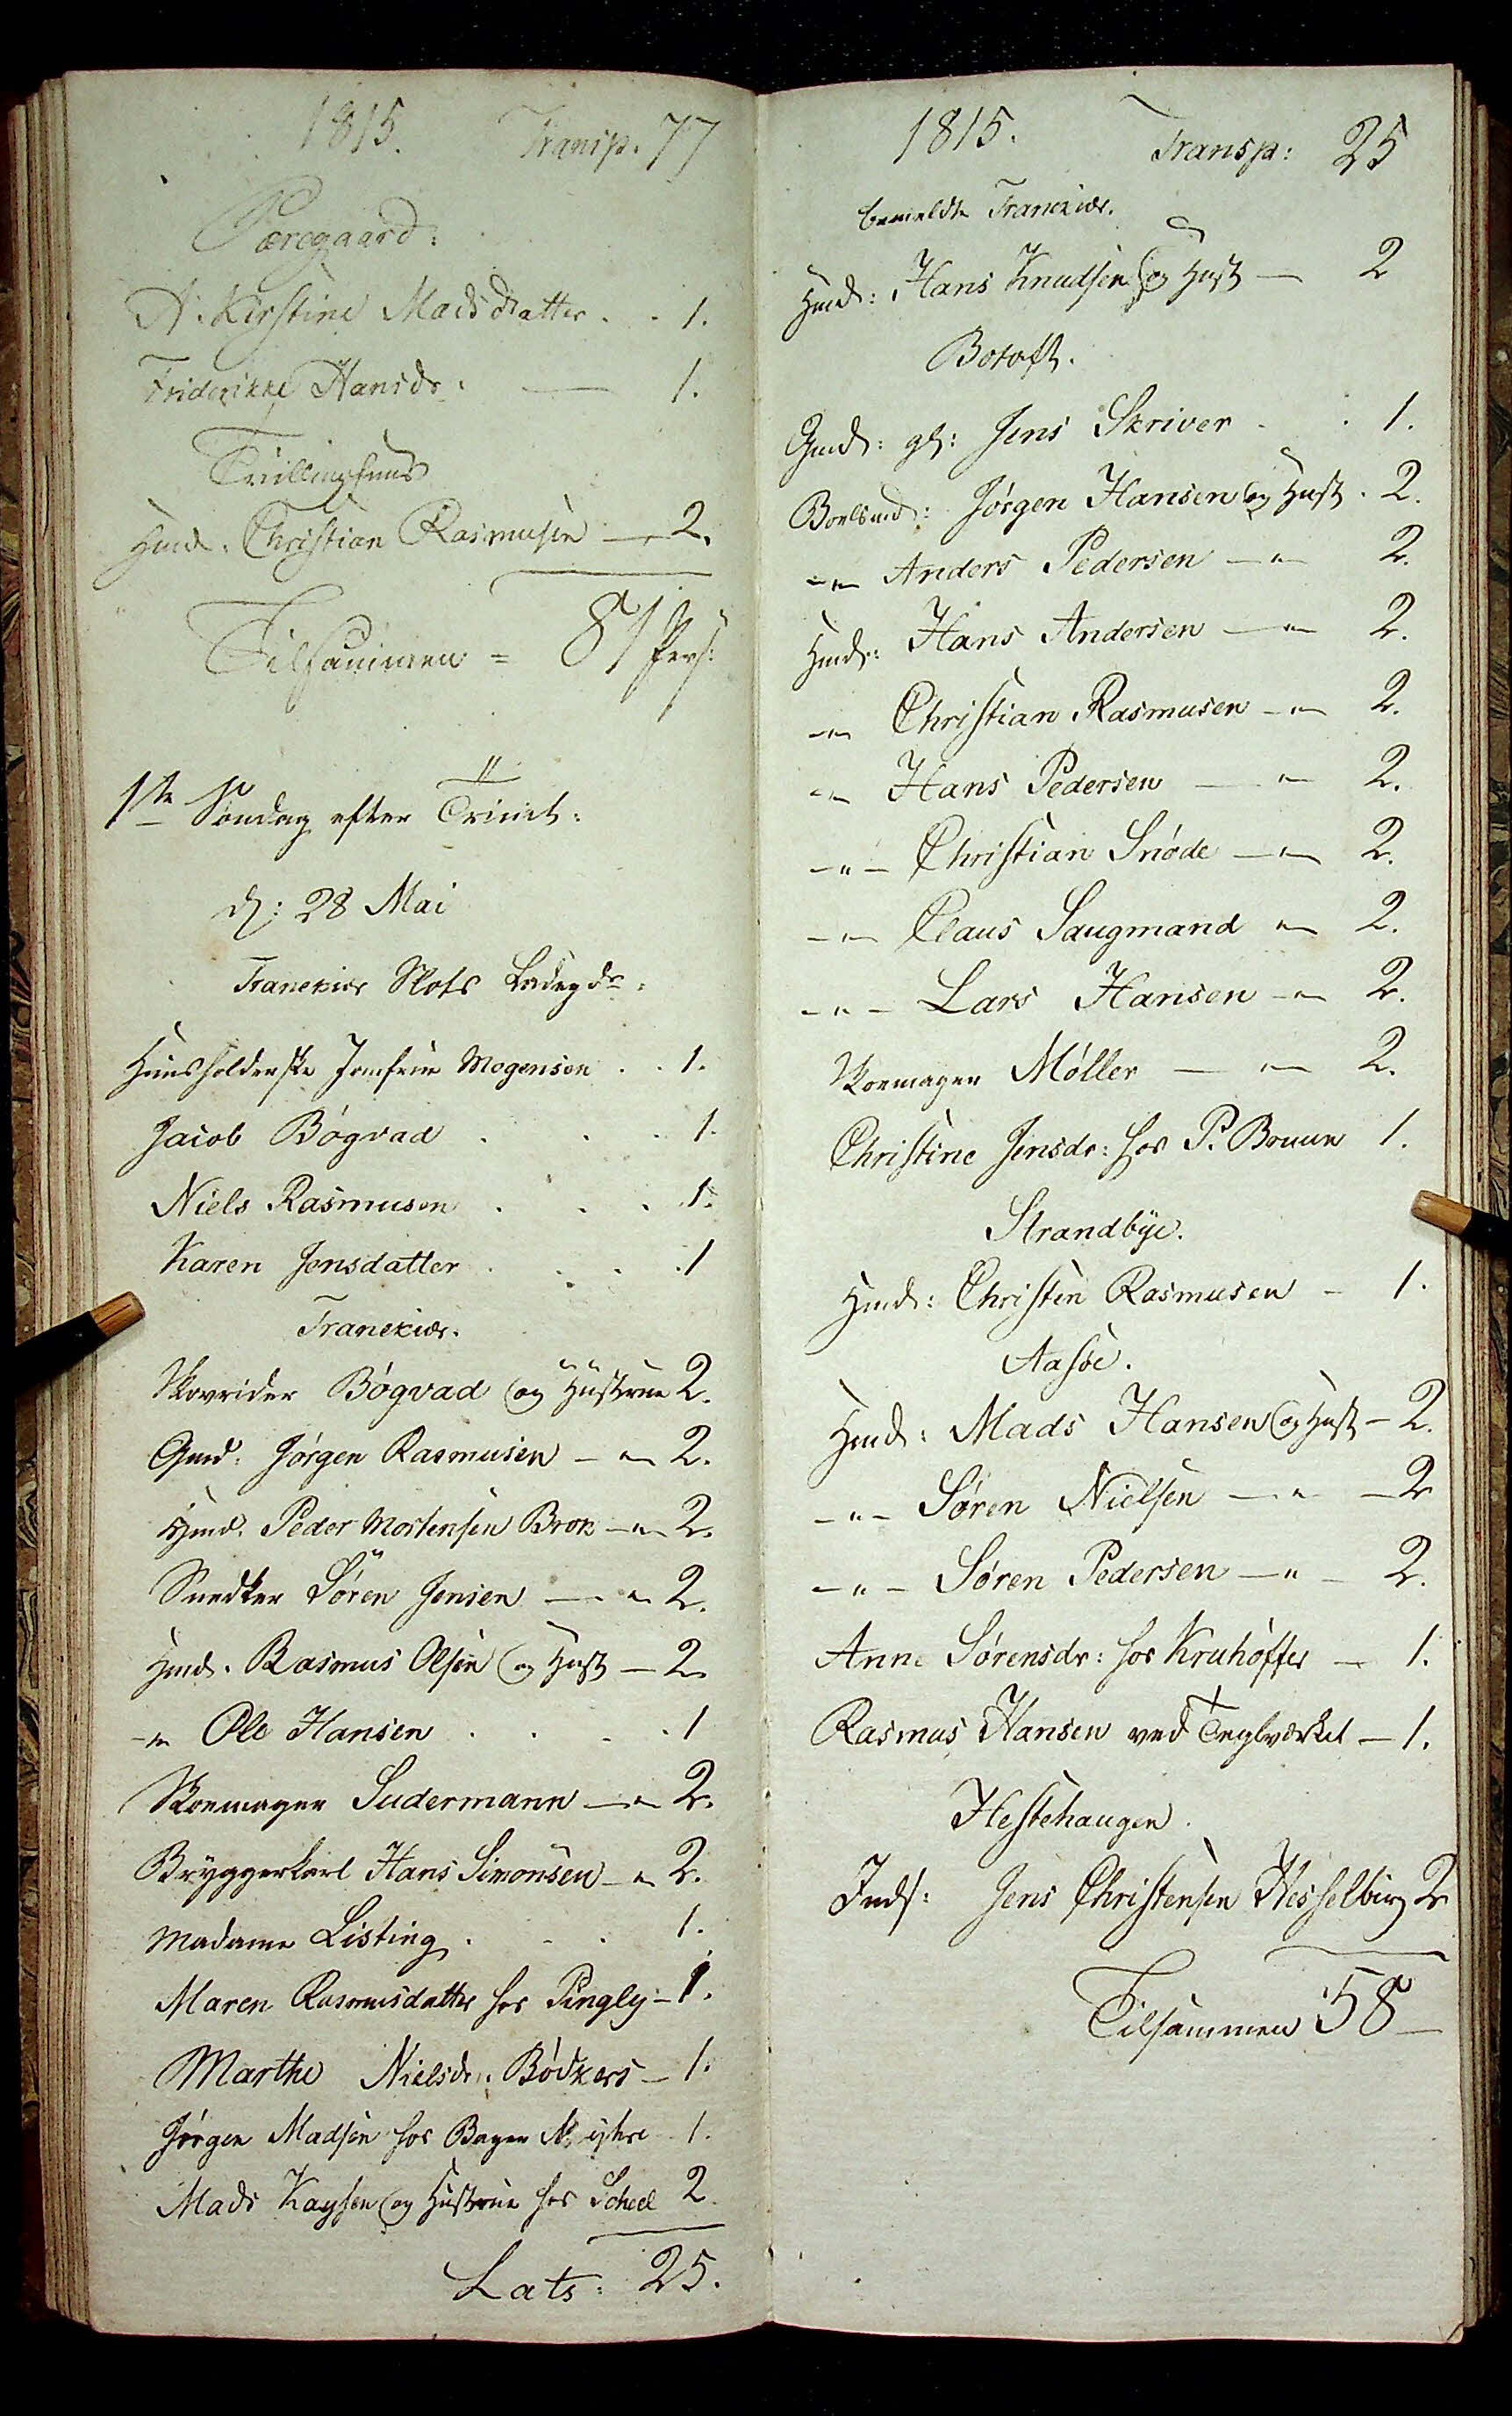1815.
Tranp: 77
Pæregaard:
A. Kirstine Madsdatter . . 1.
Friderikke Hansdr . - 1.
Tvillinghuus
Hmd: Christian Rasmusen - 2.
Tilsammen = 81 Pers:
1ste Søndag efter Trinit:
d: 28 Mai
Tranekiær Slots Ladagdr
Huusholderske Jomfrue Mogensen . . 1.
Jacob Bøgvad . . . 1.
Niels Rasmusen . . . 1.
Karen Jensdatter . . . . 1
Tranekiær.
Skovrider Bøgvad og Hustrue 2.
Gmd: Jørgen Rasmusen -"- 2.
Hmd. Peder Mortensen Brok -"- 2.
Snedker Søren Jensen -"- 2.
Hmd. Rasmus Olsen og Hust - 2.
-"- Ole Hansen . . . . 1
Skoemager Sudermann -"- 2.
Bryggerkarl Hans Simonsens -"- 2.
Madame Listing . . . 1.
Maren Rasmusdatter hos Pingly - 1.
Marthe Nielsdr Bødkers - 1.
Jørgen Madsen hos Bager Myhre . 1.
Mads Kaysen og Hustrue hos PScheel 2.
Lats: 25.
1815.
Transp: 25
bemeldte Tranekiær.
Hmd: Hans Knudsen og Hust - 2
Botoft.
Gmd: gl: Jens Skriver . .1.
Boelsmd: Jørgen Hansens og Hust . 2.
-"- Anders Pedersen -"- 2.
Hmd: Hans Andersen -"- 2.
-"- Christian Rasmusen -"- 2.
-"- Hans Pedersen -"- 2.
-"- Christian Snøde -"- 2.
-"- Claus Saugmand -"- 2.
-"- Lars Hansen -"- 2.
Skomager Møller -"- 2.
Christine Jensen hos P. Bruun 1.
Strandbye.
Hmd: Christen Rasmusen - 1
Aasøe.
Hmd: Mads Hansens og Hust - 2.
-"- Søren Nielsen -"- 2
-"- Søren Pedersen -"- 2.
Anne Sørensdr: hos Kruhøffer - 1.
Rasmus Hansen ved Teglværket - 1.
Hestehaugen
Inds: Jens Christensen Hesselbirg 2
Tilsammen 58-
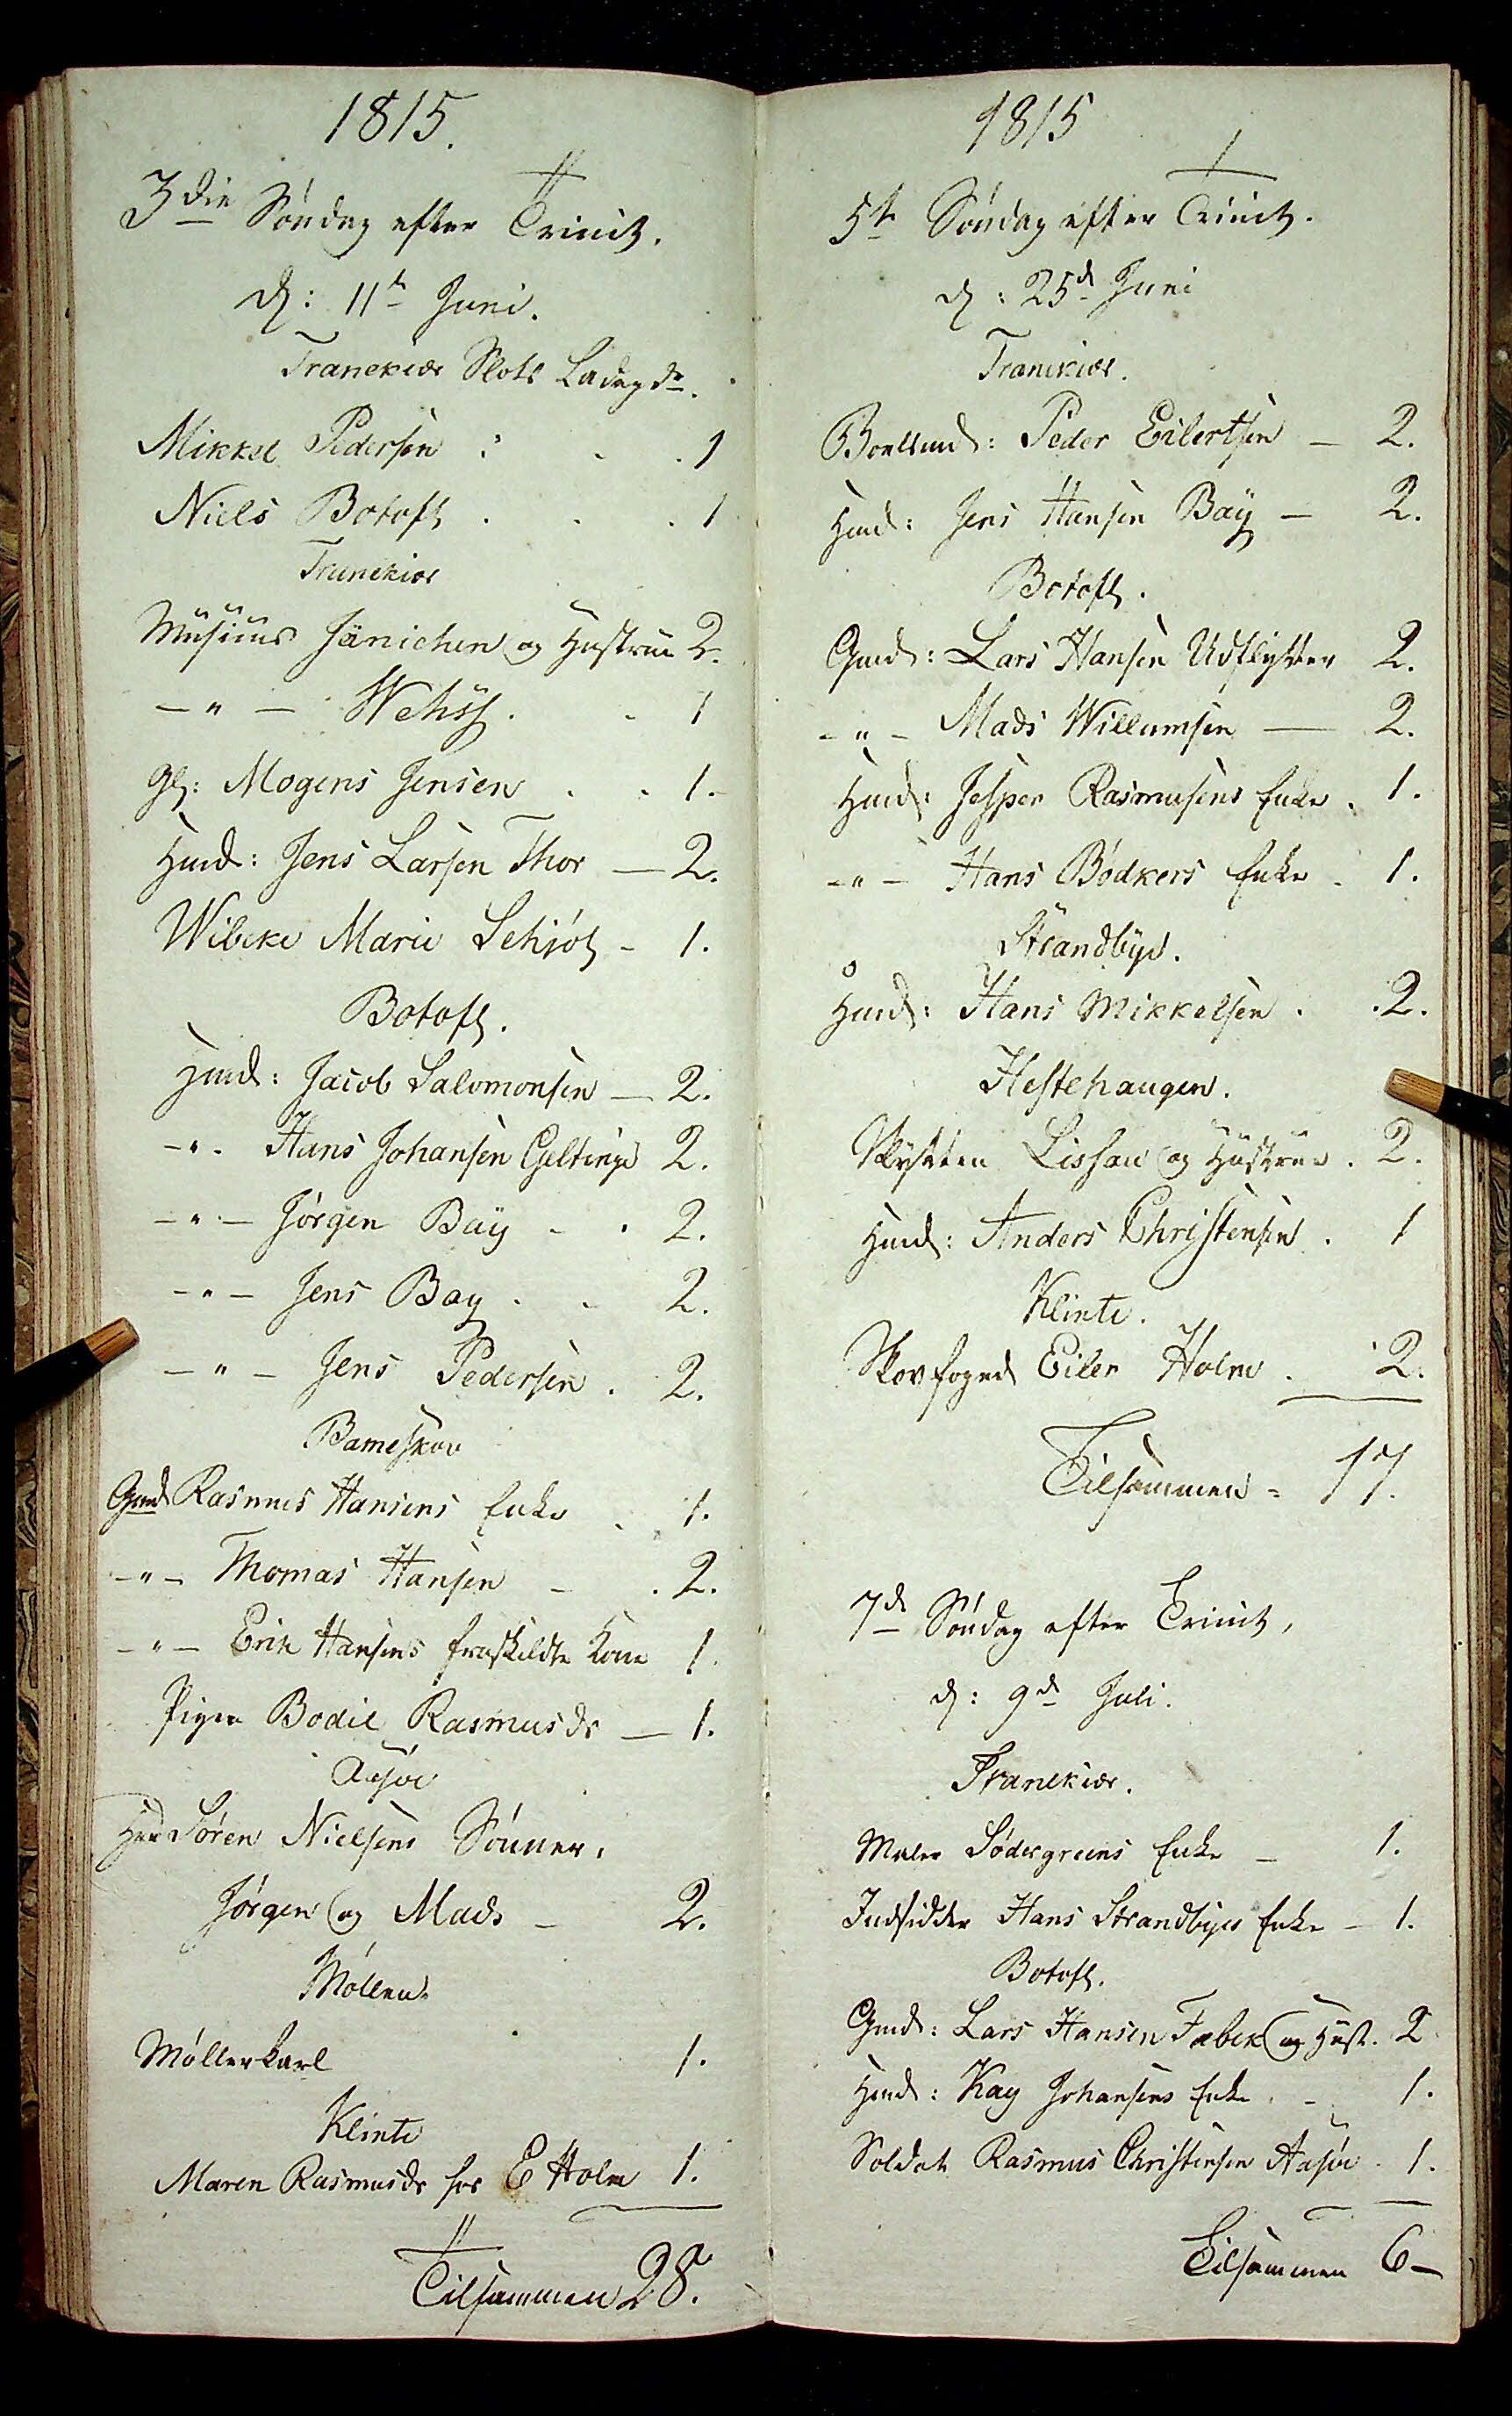1815.
3die Søndag efter Trinit.
d: 11te Juni.
Tranekiær Slots Ladegdr.
Mikkel Pedersen . . . 1
Niels Botoft . . . 1
Tranekiær
Musicus Janichen og Hustrue 2.
-"- Wehss . . 1
gl: Mogens Jensen . . 1.
Hmd: Jens Larsen Thor - 2.
Wibeke Marie Schiøt - 1.
Botoft
Hmd: Jacob Salomonsen - 2.
-"- Hans Johansen Geltinge 2.
-"- Jørgen Bay . . 2.
-"- Jens Bay . . 2.
-"- Jens Pedersen . 2.
Bameskov
Gmd Rasmus Hansens Enke . 1.
-"- Thomas Hansen - . 2.
-"- Erik Hansens fraskildte Kone 1
Pigen Bodil Rasmusdr - 1.
Aasøe.
Hmd Søren Nielsens Sønner:
Jørgen og Mads - 2.
Møllen.
Møllerkarl 1.
Klinte
Maren Rasmusdr hos E Holm 1.
Tilsammen 28.
1815
5te Søndag efter Trinit.
d: 25 Juni
Tranekiær
Boelsmd: Peder Eilertsen - 2.
Hmd: Jens Hansen Bay - 2.
Botoft
Gmd: Lars Hansen Udflytter 2.
-"- Mads Willumsen - 2.
Hmd: Jesper Rasmusens Enke . 1.
-"- Hans Bødkers Enke . 1.
Strandbye.
Hmd Hans Mikkelsen . . 2.
Hestehaugen.
Skytten Lissau og Hustrue . 2.
Hmd: Anders Christensen . 1
Klinte,
Skofoged Eiler Holm . 2.
Tilsammen = 17
7de Søndag efter Trinit,
d: 9de Juli.
Tranekiær.
Maler Sødergreens Enke - 1.
Indsidder Hans Strandbyes Enke - 1.
Bootoft.
Gmd: Lars Hansen Fæbek og Hust . 2
Hmd: Kay Johansens Enke . - 1.
Soldat Rasmus Christensen Aasøe . 1
Tilsammen 6-